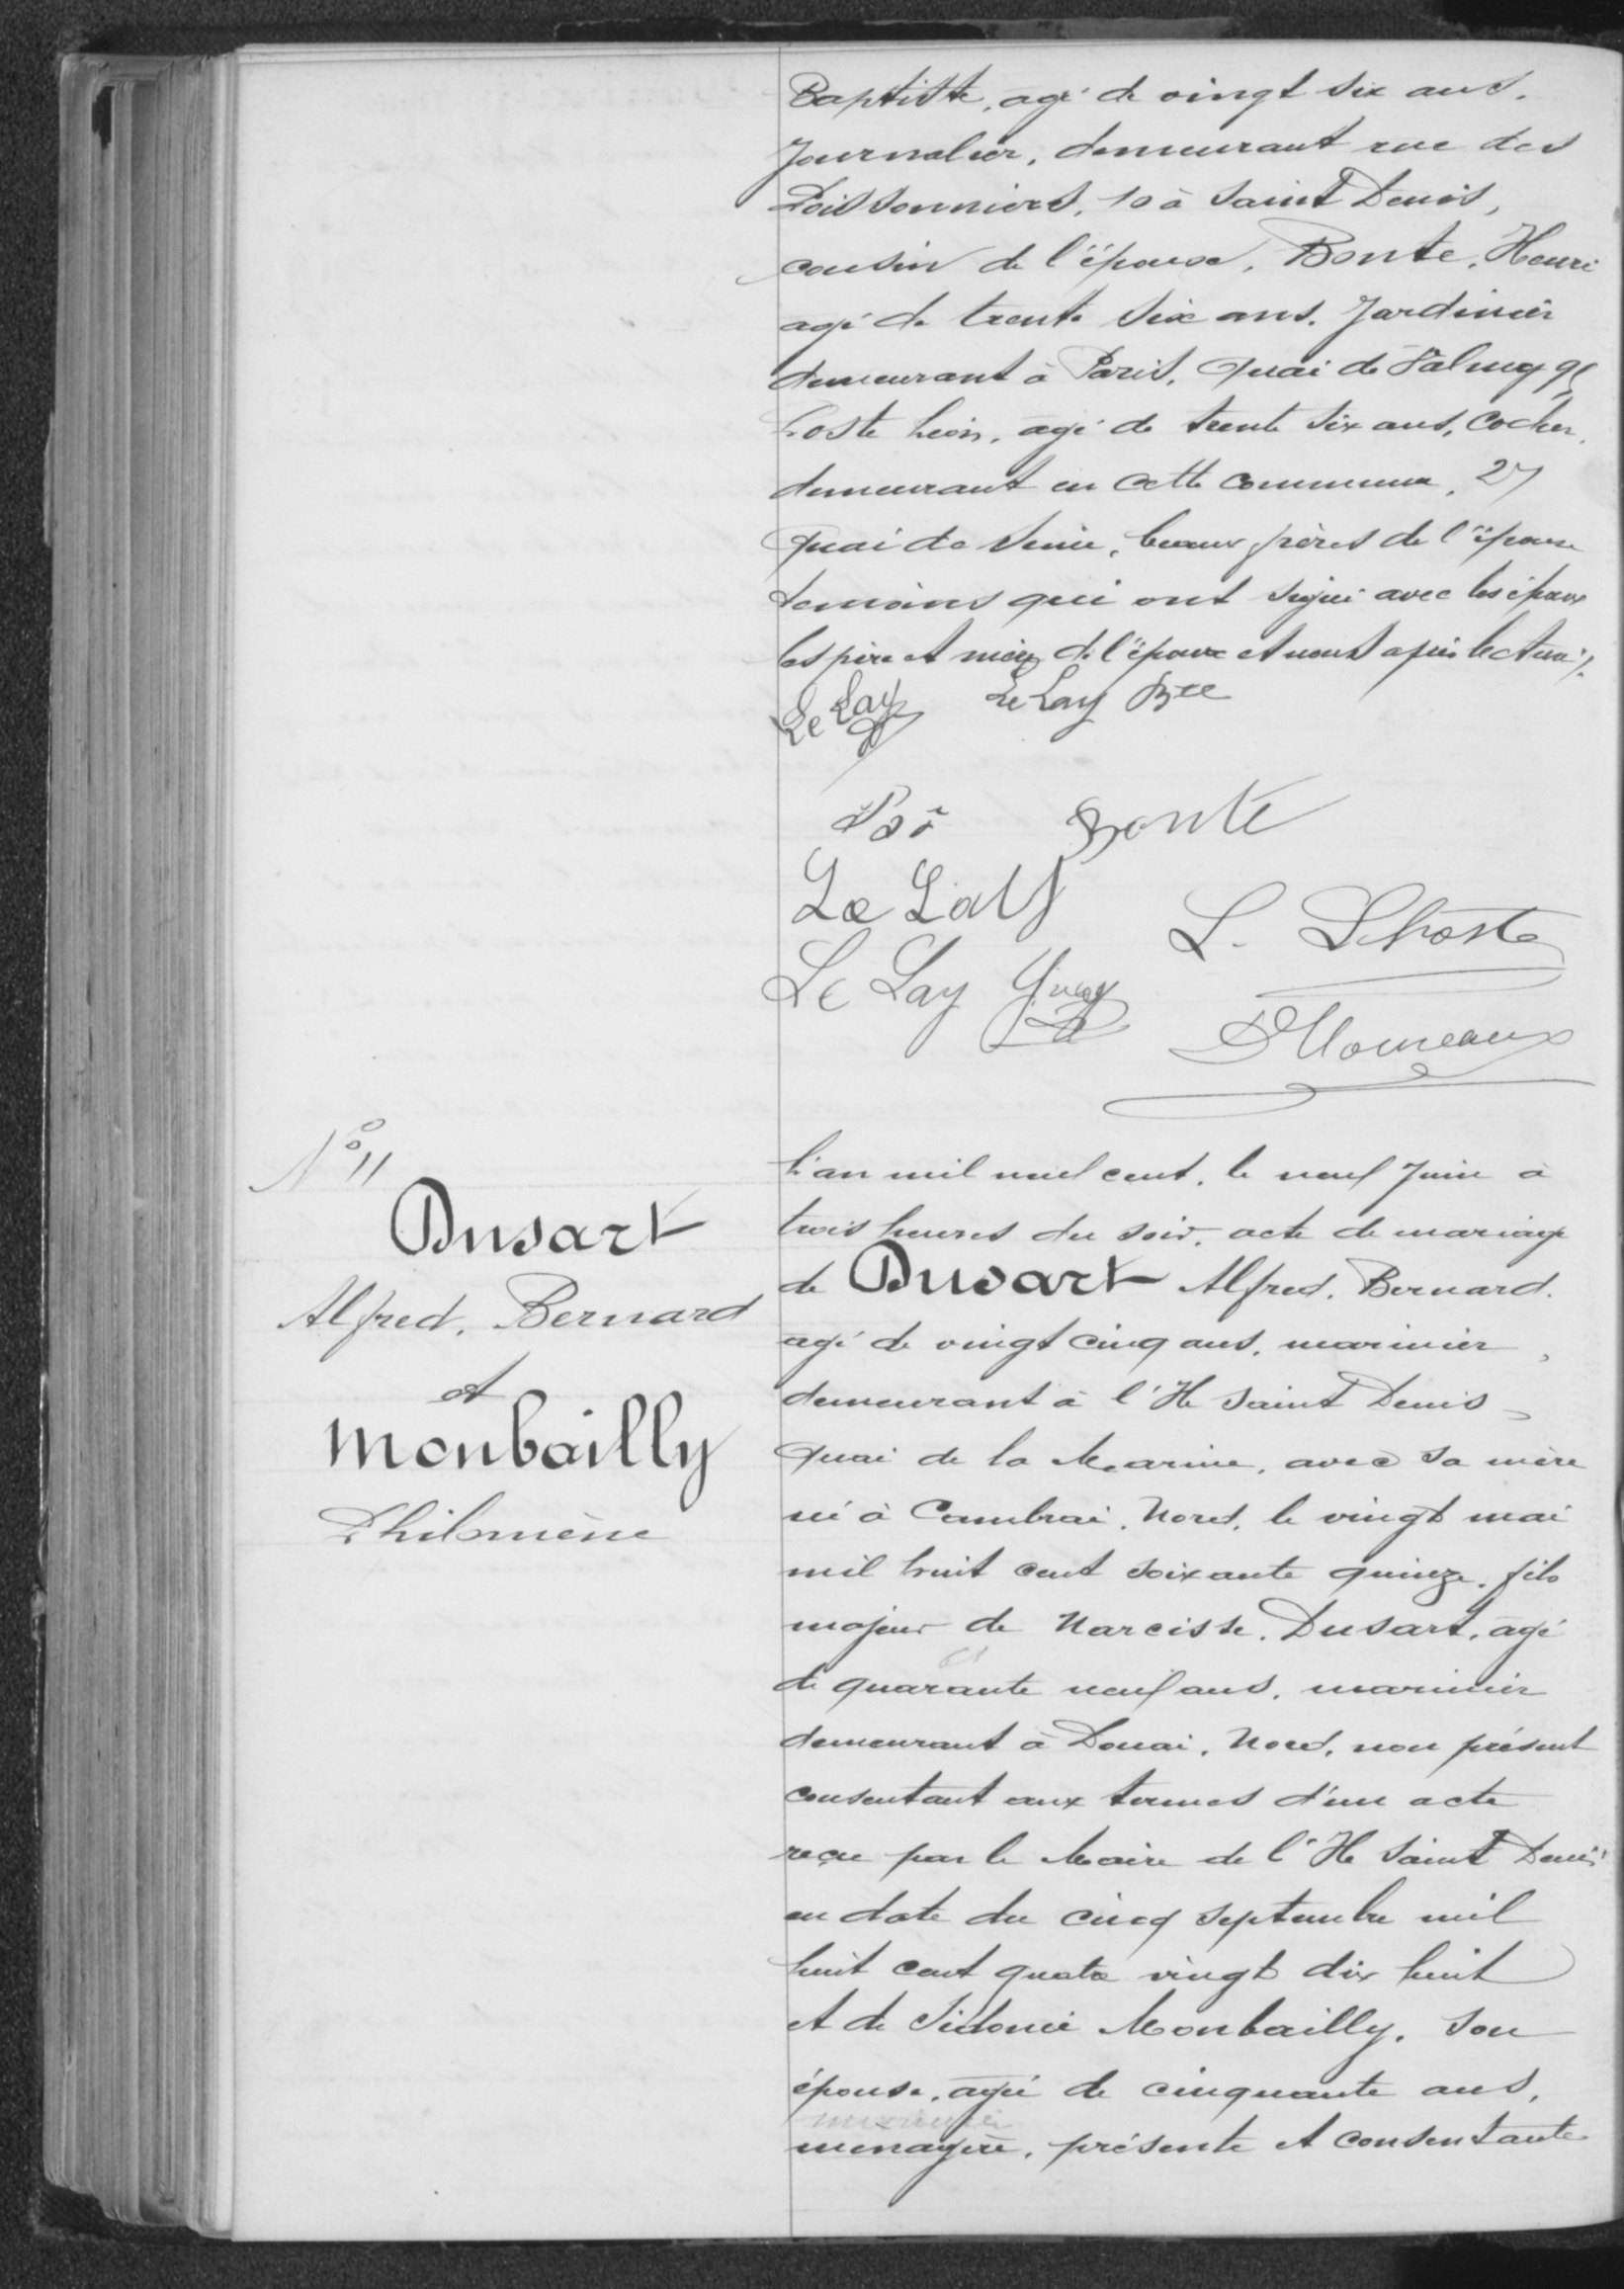Baptiste, agé de vingt six ans,
journalier, demeurant rue des
Poissonniers, 10 à Saint Denis,
cousin de l'épouse, Boute, Henri
agé de trente six ans, Jardinier
demeurant à Paris, Quai de Valuq 95
Poste Léon, âgé de trente six ans, cocher,
demeurant en cette commune, 27
Quai de Seine, beaux pères de l'épouse
témoins qui ont signé avec les époux
les père et mère de l'époux et nous après lecture./.
N°11
Dusart
Alfred Bernard
et
Monbailly
Philomène
l'an mil neuf cent, le neuf Juin à
trois heures du soir, acte de mariage
de Duvart Alfred, Bernard
agé de vingt cinq ans, marinier,
demeurant à L'Ile Saint Denis,
Quai de la Marine, avec sa mère
né à Cambrai, Nord, le vingt mai
mil huit cent soixante quinze fils
majeur de Narcisse Dusart, agé
de quarante neuf ans, marinier
demeurant à Douai, Nord, non présent
consentant aux termes d'un acte
reçu par le Maire de L'Ile Saint Denis
en date du cinq septembre mil
huit cent quatre vingt dix huit
et de Sidonie Monbailly, son
épouse, âgée de cinquante ans,
ménagère, présente et consentante
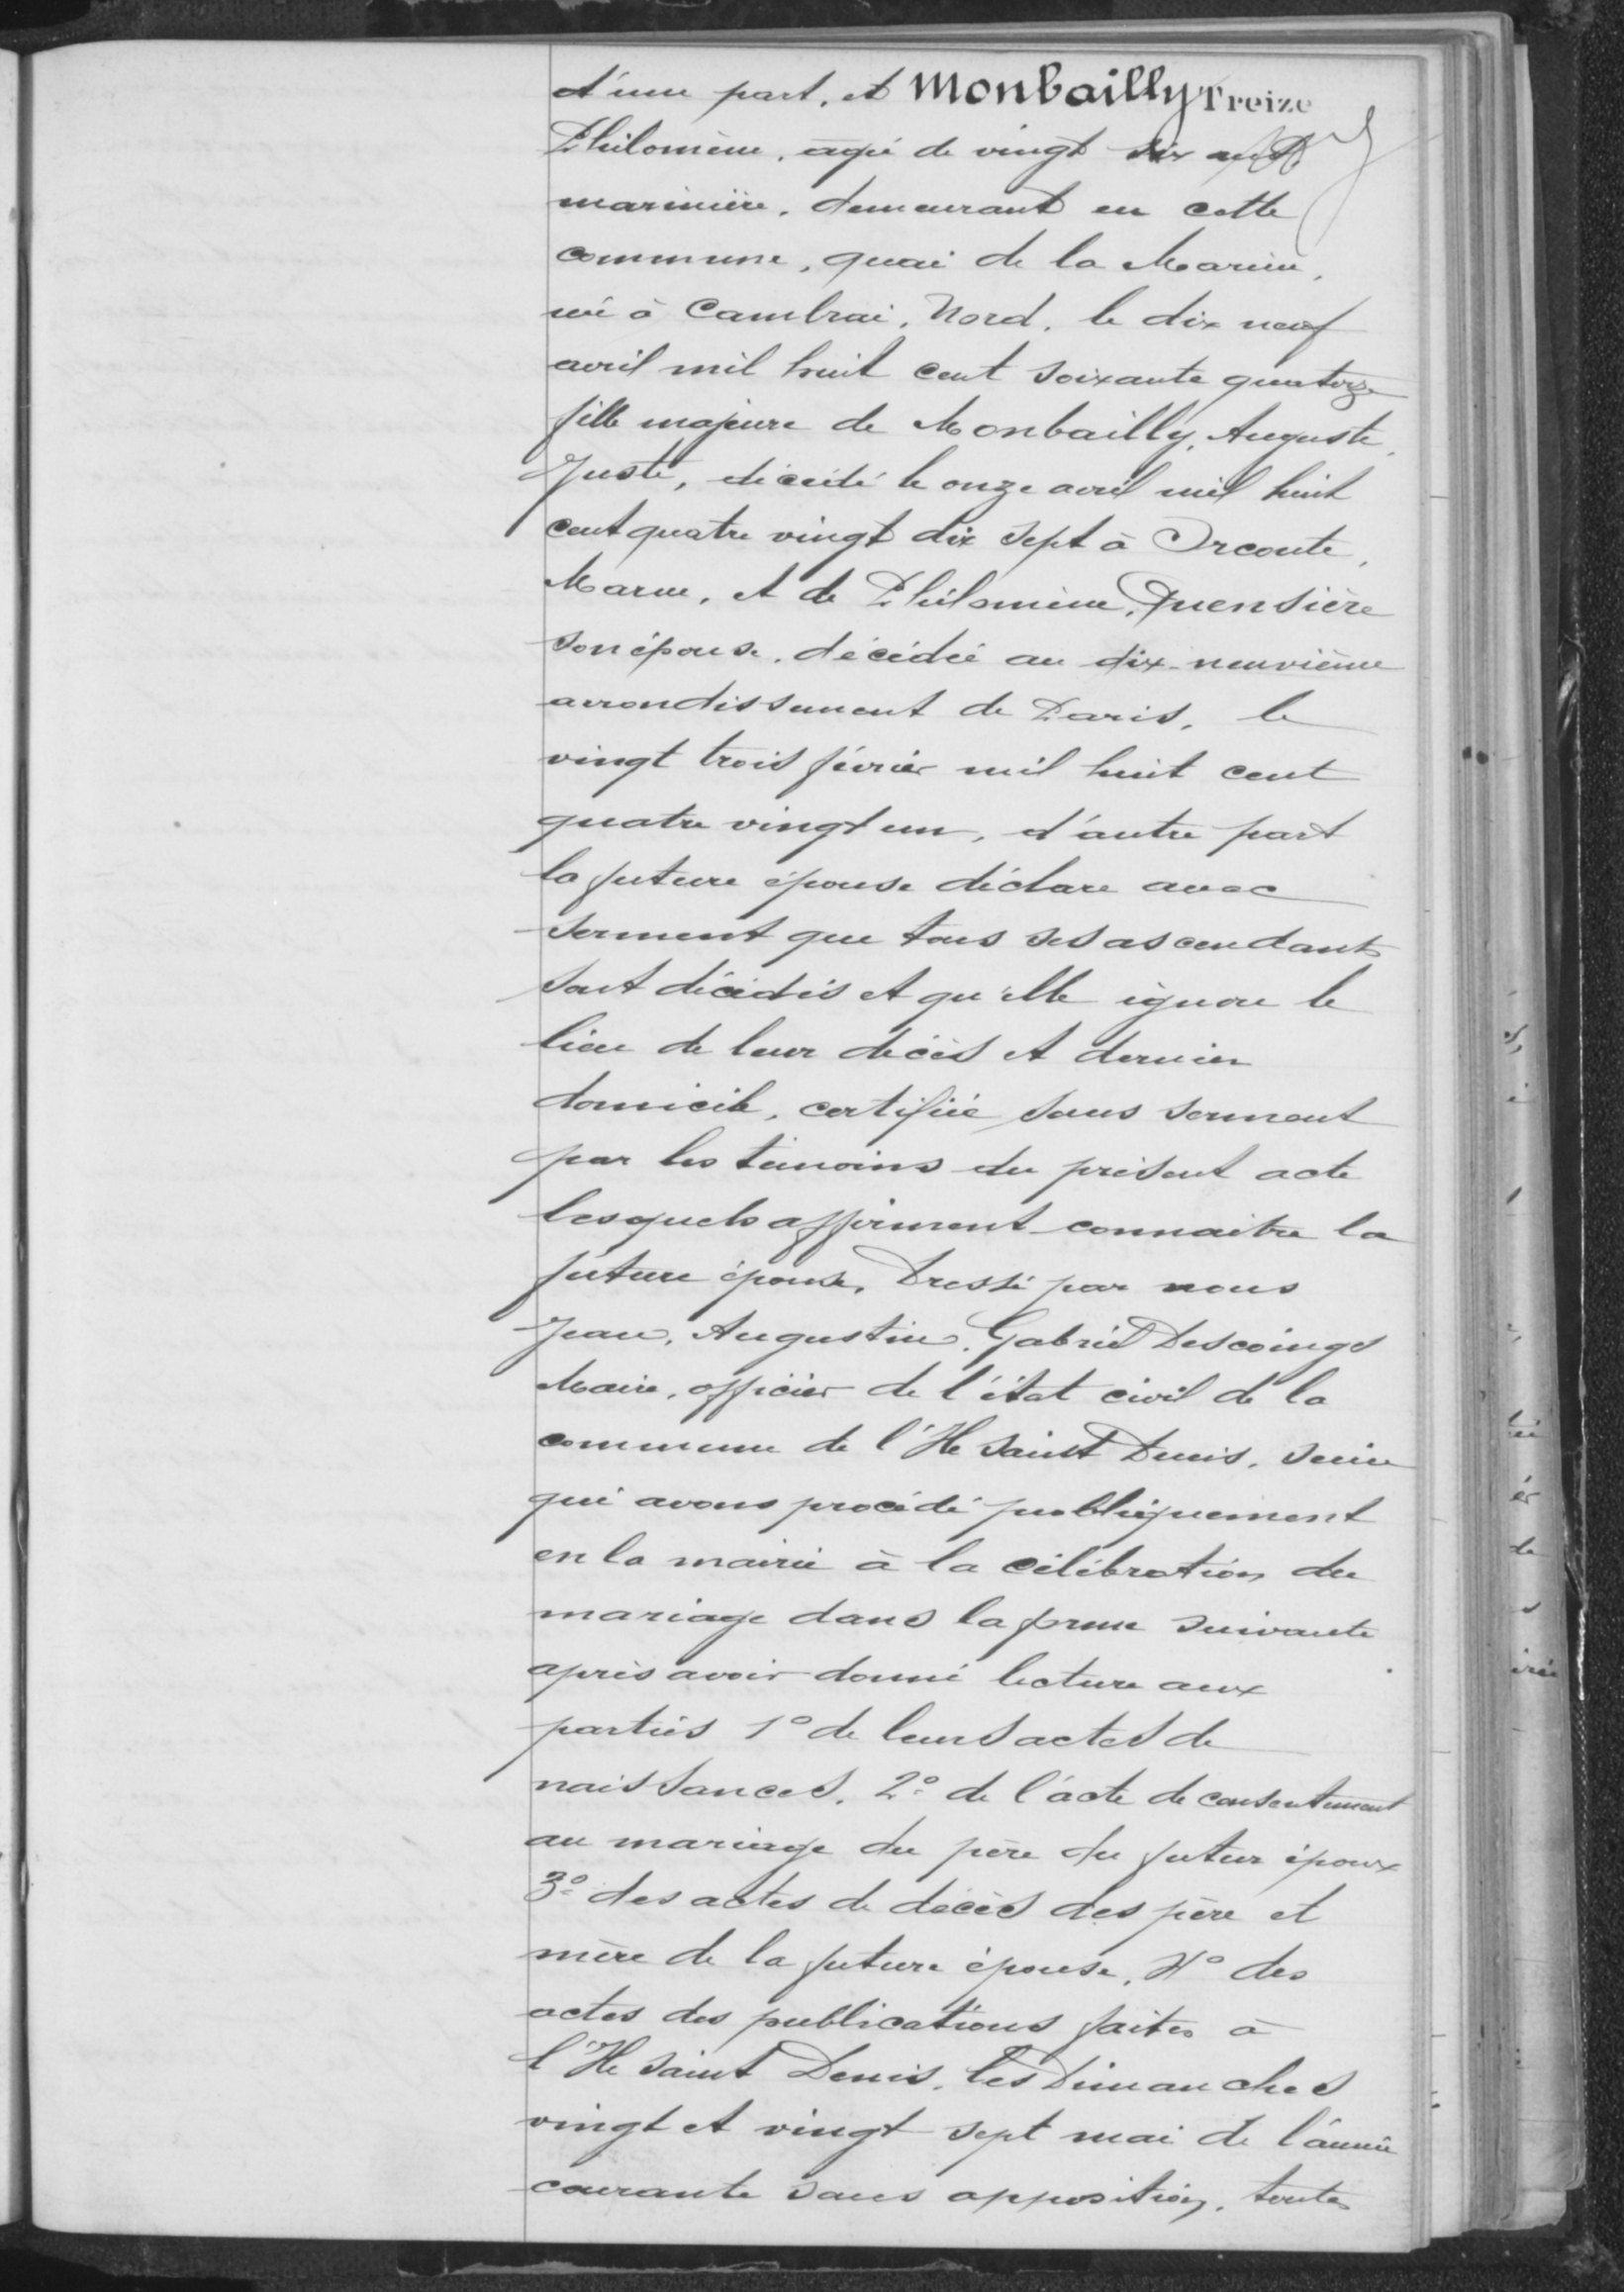d'une part, et Monbailly Treize
Philomène, agée de vingt six ans,
marinière, demeurant en cette
commune, quai de la Marine,
née à Cambrai, Nord, le dix neuf
avril mil huit cent soixante quatorze
fille majeure de Monbailly, Auguste
Juste, décédé le onze avril mil huit
cent quatre vingt dix sept à Orcoute,
Marne, et de Philomène, Quensière
son épouse, décédée au dix-neuvième
arrondissement de Paris le
vingt trois février mil huit cent
quatre vingt un, d'autre part
la futur épouse déclare avec
serment que tous ses ascendants
sont décédés et qu'elle ignore le
lieu de leur décès et dernier
domicile, certifiée sous serment
par les témoins du présent acte
lesquels affirment connaitre la
future épouse, Dressé par nous
Jean, Augustin, Gabriel Descoinge
Maire, officier de l'état civil de la
commune de l'Ile Saint Denis, Seine
qui avons procédé publiquement
en la mairie à la célébration du
mariage dans la preuve suivante
après avoir donné lecture aux
parties 1° de leurs actes de
naissances, 2° de l'acte de consentement
au mariage du père du futur époux
3°: des actes de décès des père et
mère de la future épouse, 4° des
actes des publications faites à
l'Ile Saint Denis, les dimanches
vingt et vingt sept mai de l'année
courante sans opposition, toutes